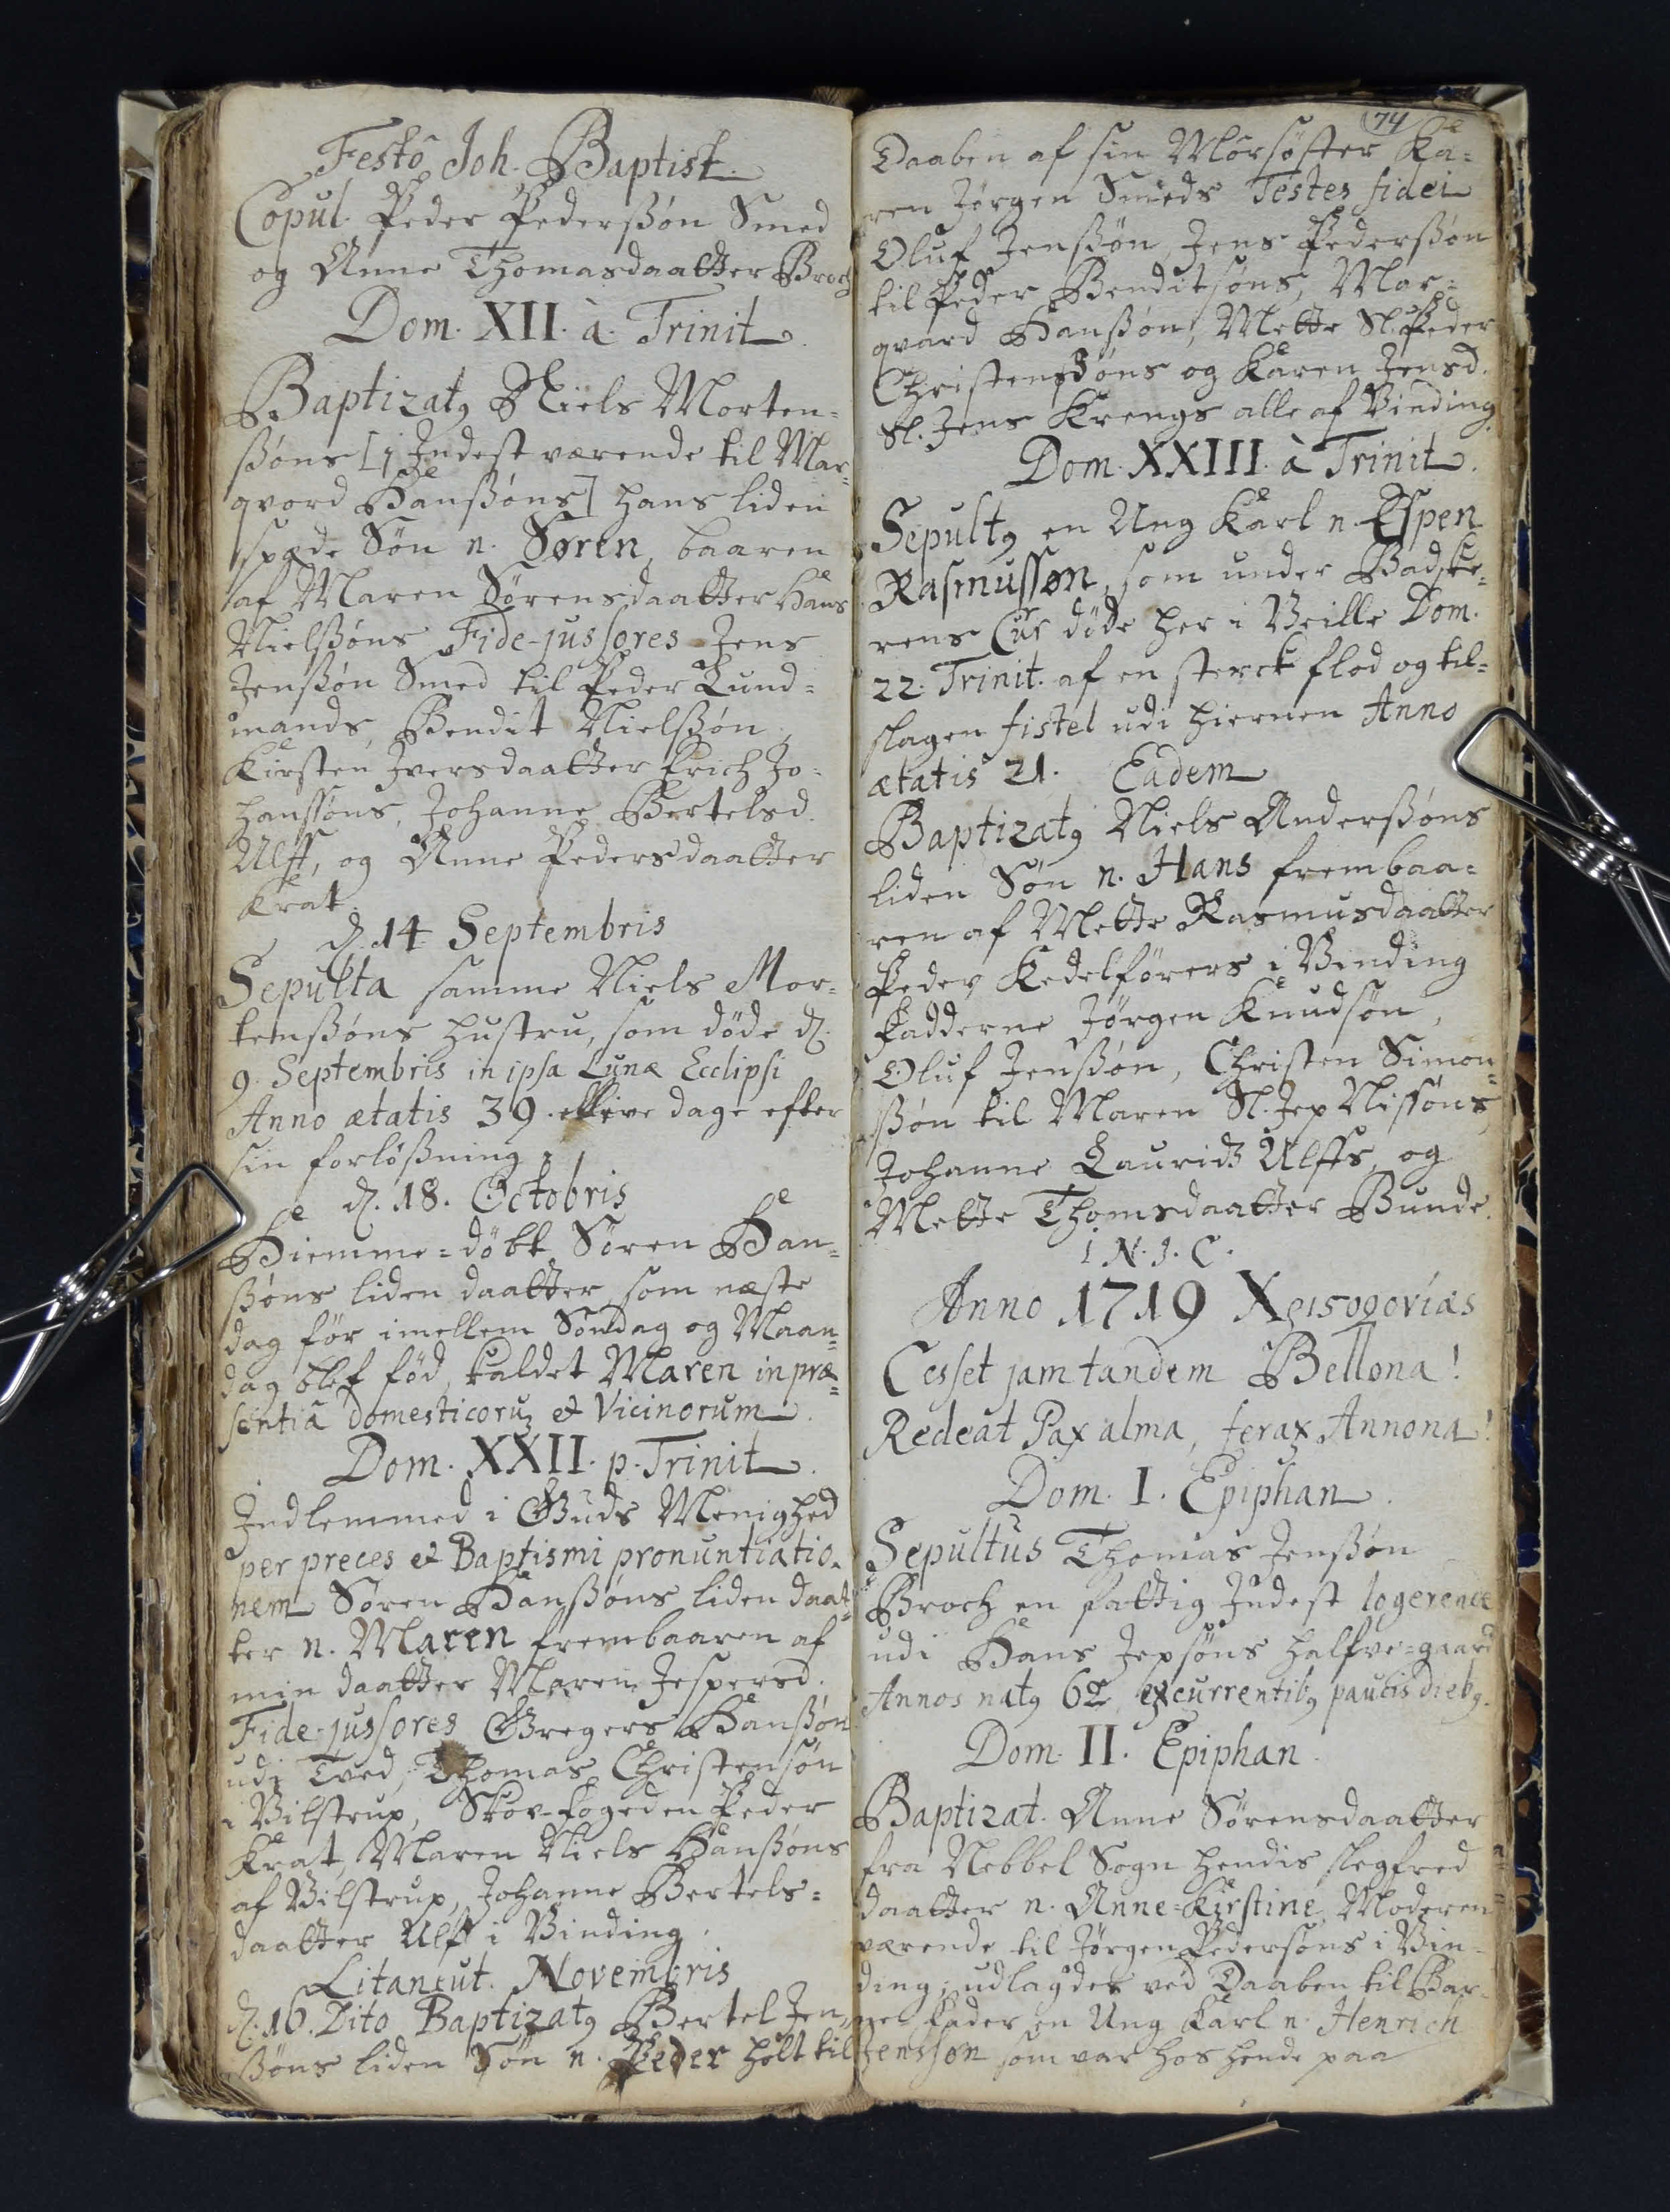Festo Joh. Baptist.
Copul. Peder Pederssøn Smed
og Anne Thomasdaatter Broch
Dom. XII. a. Trinit
Baptizatq Niels Morten¬
ssøns [1 Indest værende til Mar¬
qvard Hanssøns] hans liden
spæde Søn n. Søren, baaren
af Maren Sørensdaatter Hans
Nielssøns Fide-jussores Jens
Jenssøn Smed til Peder Lund¬
mands, Bendit Nielssøn
Kirsten Iversdaatter Erich Jo¬
hanssøns, Johanne Bertelsd
Ulff, og Anne Pedersdaatter
Krat
d. 14 Septembris
Sepulta samme Niels Mor¬
tenssøns hustru, som døde d.
9. Septembris in ipsa Lunæ Ecclipsi
Anno ætatis 39 elleve dage efter 
sin forløssning
d. 18. Octobris
Hiemme=døbt Søren Han¬
ssøns liden daatter, som næste
dag før imellem Søndag og Maan¬
dag blef fød, kaldet Maren, in præ¬
sentia domesticoruz  et Vicinorum
Dom. XXII. p. Trinit
Indlemmed i Guds Menighed
per preces et Baptismi pronuntiatio
nem Søren Hanssøns liden daat¬
ter n. Maren frembaaren af
min daatter Maren Jespersd.
Fide-jussores Gregers Hanssøn
udi Tved, Thomas Christensøn
i Vilstrup, Skof-fogeden Peder
Krat, Maren Niels Hanssøns
af Vilstrup, Johanne Bertels¬
daatter Ulf i Vinding
Litaneut. Novembris
d. 16. Dito Baptizatq Bertel Jen¬
asøns liden Søn n. Peder holdt til
74
Daaben af sin Morsøster Ka¬
ren Jørgen Smeds Testes fidei
Oluf Jenssøn, Jens Pederssøn
til Peder Benditsøns, Mar¬
qvard Hanssøn, Mette Sl. Peder
Christenssøns og Karen Jensd.
Sl. Jens Krengs alle af Vinding
Dom. XXIII. a Trinit
Sepultq en Ung Karl n Espen
Rasmussøn, som under Badske¬
rens Cur døde her i Veille Dom.
22: Trinit. af en sterck flod og til¬
slagen fistel udi hiernen Anno 
ætatis 21.
Eadem
Baptizatq Niels Anderssøns
liden Søn n. Hans, frembaa¬
ren af Mette Rasmusdaatter
Peder Kedelførers i Vinding
Fadderne Jørgen Knudsøn
Oluf Jenssøn, Christen Simon¬
ssøn til Maren Sl. Jep Nissøns
Johanne Lauridz Ulffs, og
Mette Thomasdaatter Bunde.
I N. J. C.
Anno 1719 X##ovias
Cesset jam tandem Bellona
Redeat Paxalma, ferax Annona
Dom. I Epiphan
Sepultus Thomas Jenssøn
Broch en fattig Indest logerende 
udi Hans Jepsøns halfve=gaard
Annos natq 62 exeurrentibq paucisdiebq
Dom. II. Epiphan
Baptizat. Anne Sørensdaatter
fra Nebbel Sogn hendis slegfred
daatter n. Anne=Kirstine, Moderen
værende til Jørgen Pedersøns i Vin¬
ding, udlagdes ved Daaben til Bar¬
ne=Fader en Ung Karl n. Henrich
Jenssøn, som var hos hende paa
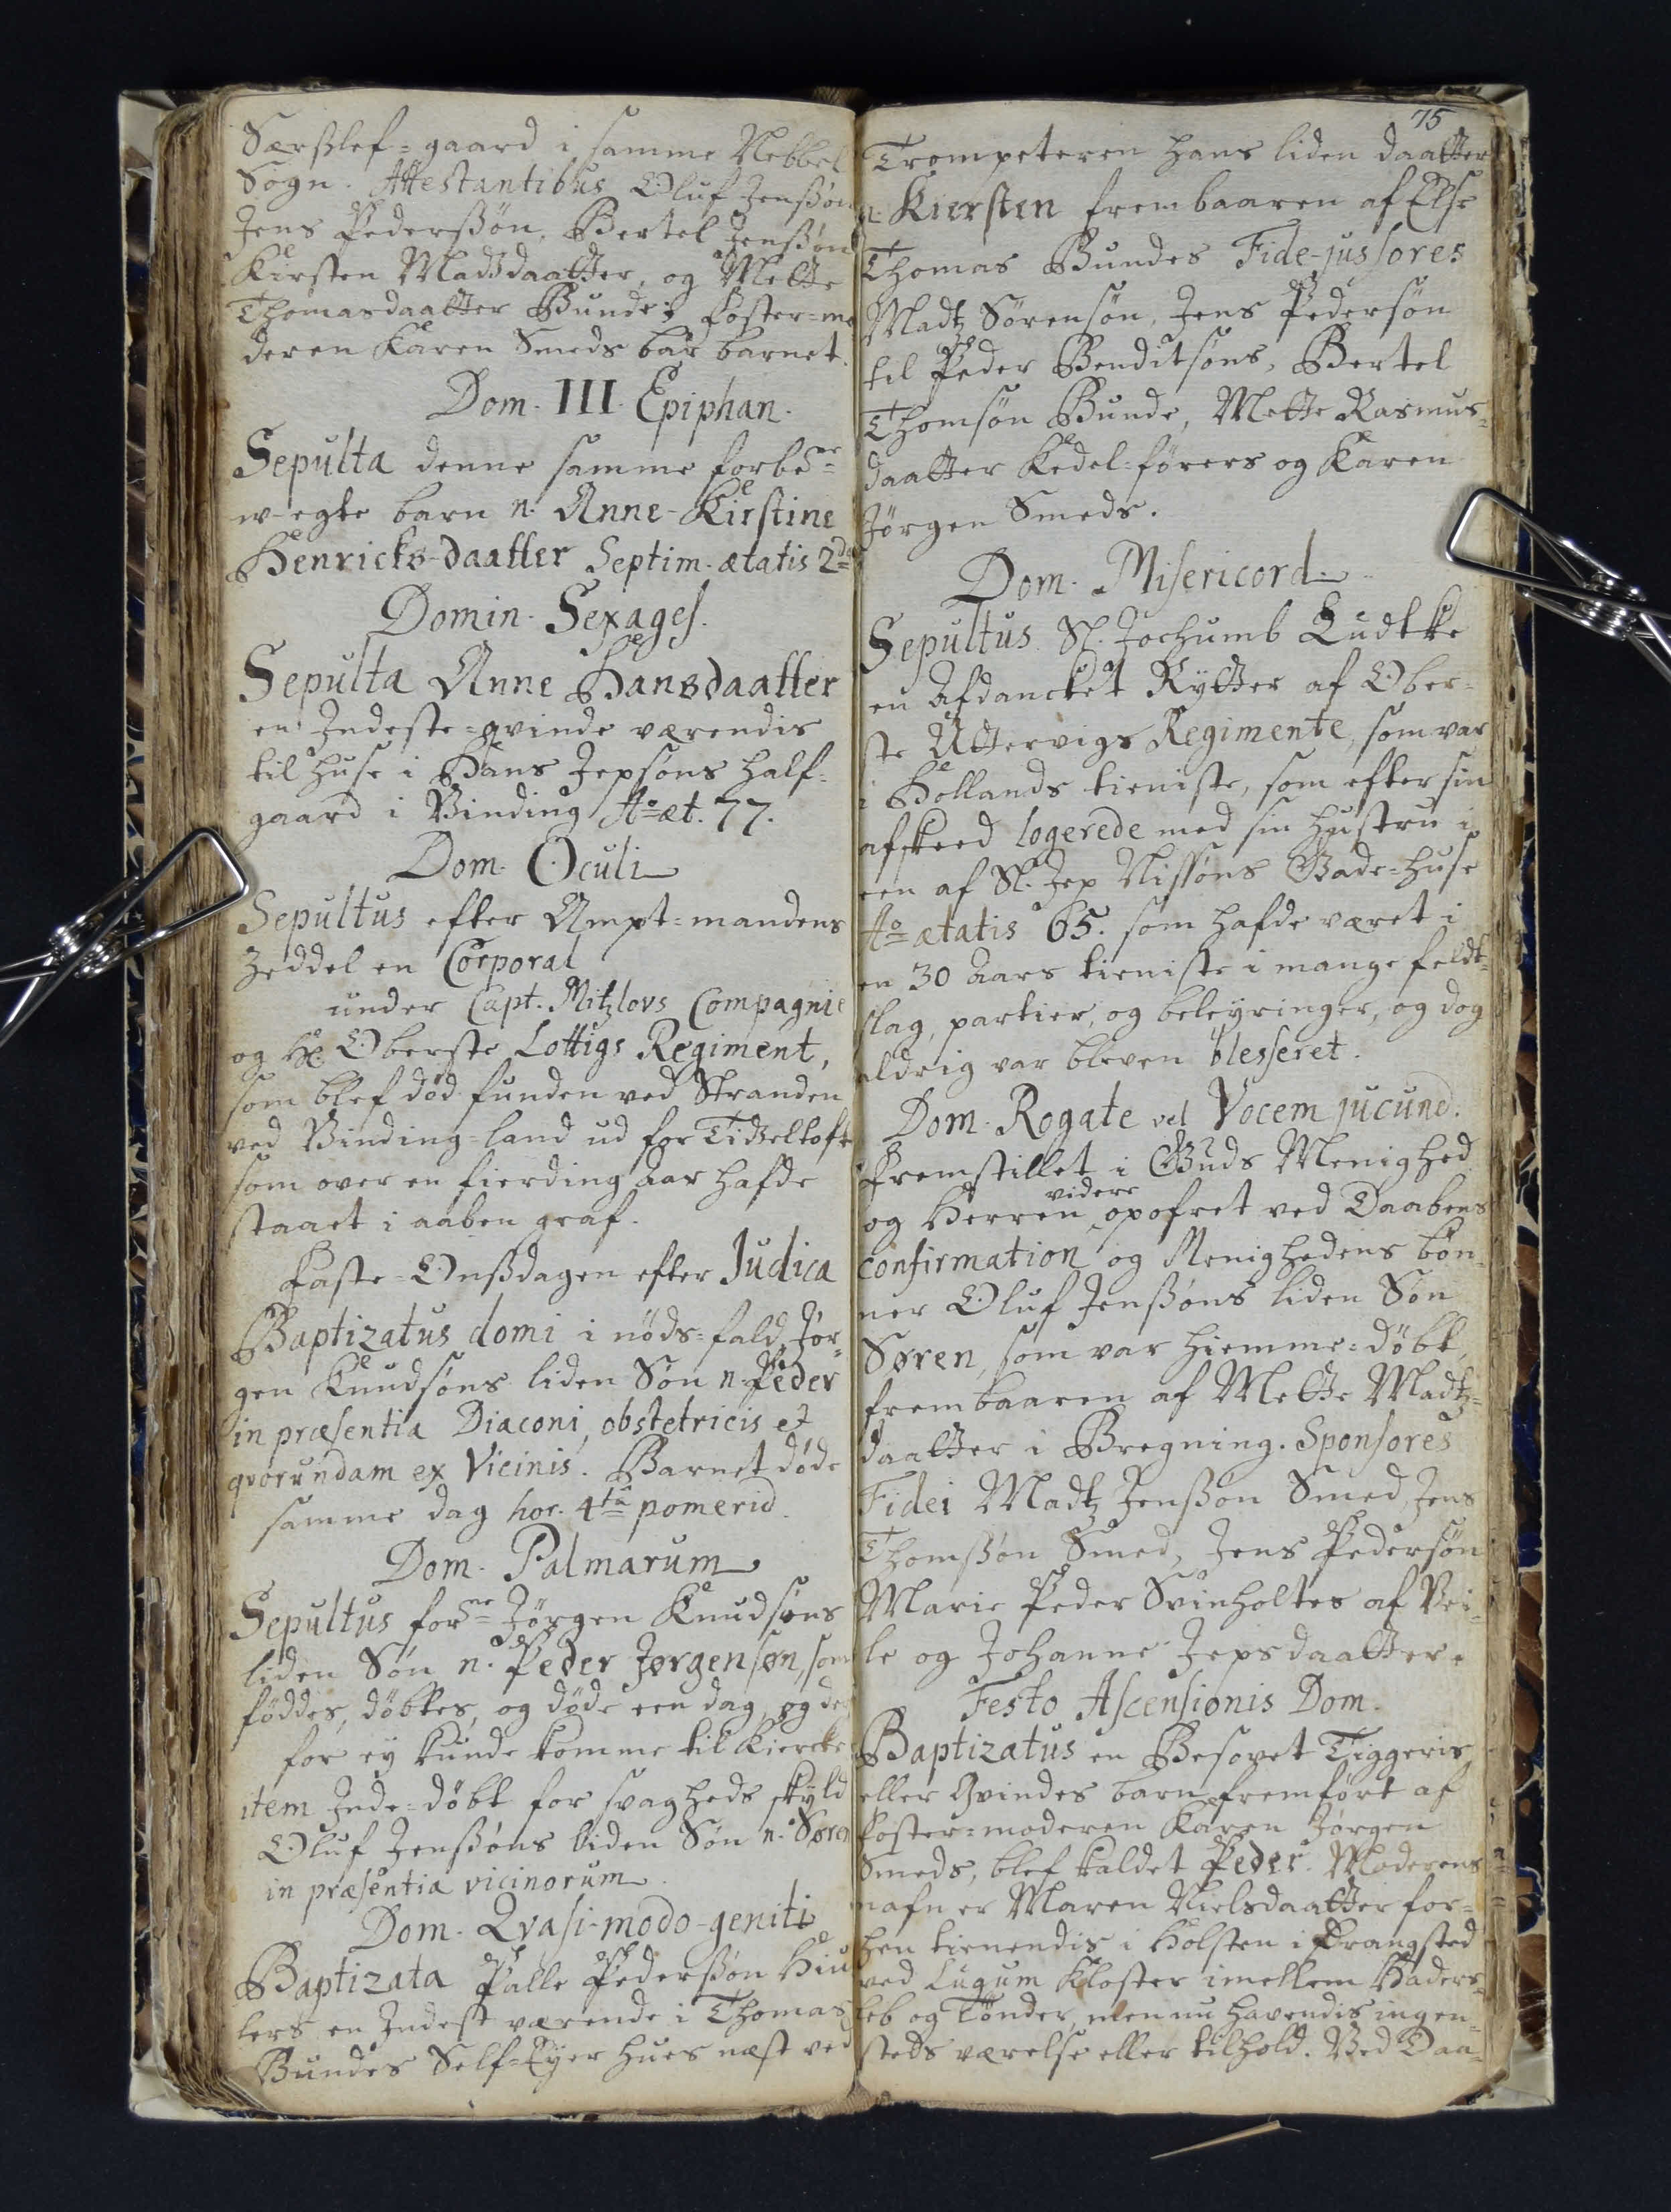Særsslef=gaard i samme Nebbel
Sogn. Attestantibus Oluf Jenssøn
Jens Pederssøn, Bertel Jensøn
Kirsten Madtzdaatter, og Mette
Thomasdaatter Bunde; Foster=mo
deren Karen Smeds bar barnet
Dom. III. Epiphan.
Sepulta denne samme forbene
w-egte barn n. Anne-Kirstine
Henrichs-daatter Septim. ætatis 2d##
Domin Sexages.
Sepulta Anne Hansdaatter
en Indeste=qvinde værendis
til huse i Hans Jepsøns half¬
gaard i Vinding Ao æt. 77.
Dom. Oculi.
Sepultus efter Ampt=mandens 
Zeddel en Corporal 
under Capt. Mitzlovs Compagnie
og Hl Oberste Lottigs Regiment
som blef død funden ved Stranden
ved Vinding=Land ud for Tidzeltoft
som over en fierding Aar hafde
staaet i aaben graf.
Faste=Onssdagen efter Judica
Baptizatus domi i nøds=fald Jør¬
gen Knudsøns liden Søn n. Peder
in præsentia Diaconi obstetricis et
qvorundam ex Vieinis. Barnet døde
samme dag hoe. 4ta pomerid
Dom. Palmarum
Sepultus forne Jørgen Knudsøns
liden Søn n. Peder Jørgensøn, som
føddis, døbtes, og døde een dag, og der
for ey kunde komme til Kiercke
item Inde=døbt for svagheds skyld
Oluf Jenssøns liden Søn n. Søren
in præsentia vicinorum
Dom. Qvasi-modo-geniti
Baptizata Palle Pederssøn Hiu
lers en Indest værende i Thomas
Bundes Self=Eyer Huus næst ved
75
Trompeteren hans liden daatter
n. Kiersten, frembaaren af Else
Thomas Bundes Fide-jussores
Madtz Sørensøn, Jens Pederssøn
til Peder Benditsøns, Bertel
Thomsøn Bunde, Mette Rasmus¬
daatter Kedel=førers og Karen 
Jørgen Smeds.
Dom. Misericord.
Sepultus Sl. Jochumb Ludtke
en Afdancket Rytter af Ober¬
ste Uttervigs Regimente, som var
i Hollands tieneste, som efter sin
afskeed logerede med sin hustru i
een af Sl. Jep Nissøns Gade=huse
Ao ætatis 65. som hafde været i
en 30 Aars tieneste i mange feldt¬
slag, partier, og beleyringer, og dog
aldrig var bleven blesseret
Dom. Rogate vel Vocem jucund.
Fremstillet i Guds Menighed
videre
og Herren opofret ved Daabens
confirmation og Menighedens bøn¬
ner Oluf Jenssøns liden Søn
Søren, som var hiemme=døbt
frembaaren af Mette Madtz¬
daatter i Breining. Sponsores
Fidei Madtz Jenssøn Smed, Jens
Thomssøn Smed, Jens Pedersøn
Marie Peder Svinholtes af Vei¬
le og Johanne Jepsdaatter.
Festo Ascensionis Dom.
Baptizatus en Besovet Tiggeris 
eller Qvindes barn fremført af
Foster=moderen Karen Jørgen
Smeds, blef kaldet Peder. Moderens 
Nafn er Maren Nielsdaatter for¬
hen tienendis i Holsten i ##rangsted
ved Lugum Kloster imellem Haders¬
leb og Tønder men nu havendis ingen¬
steds værelse eller tilhold. Ved Daa¬